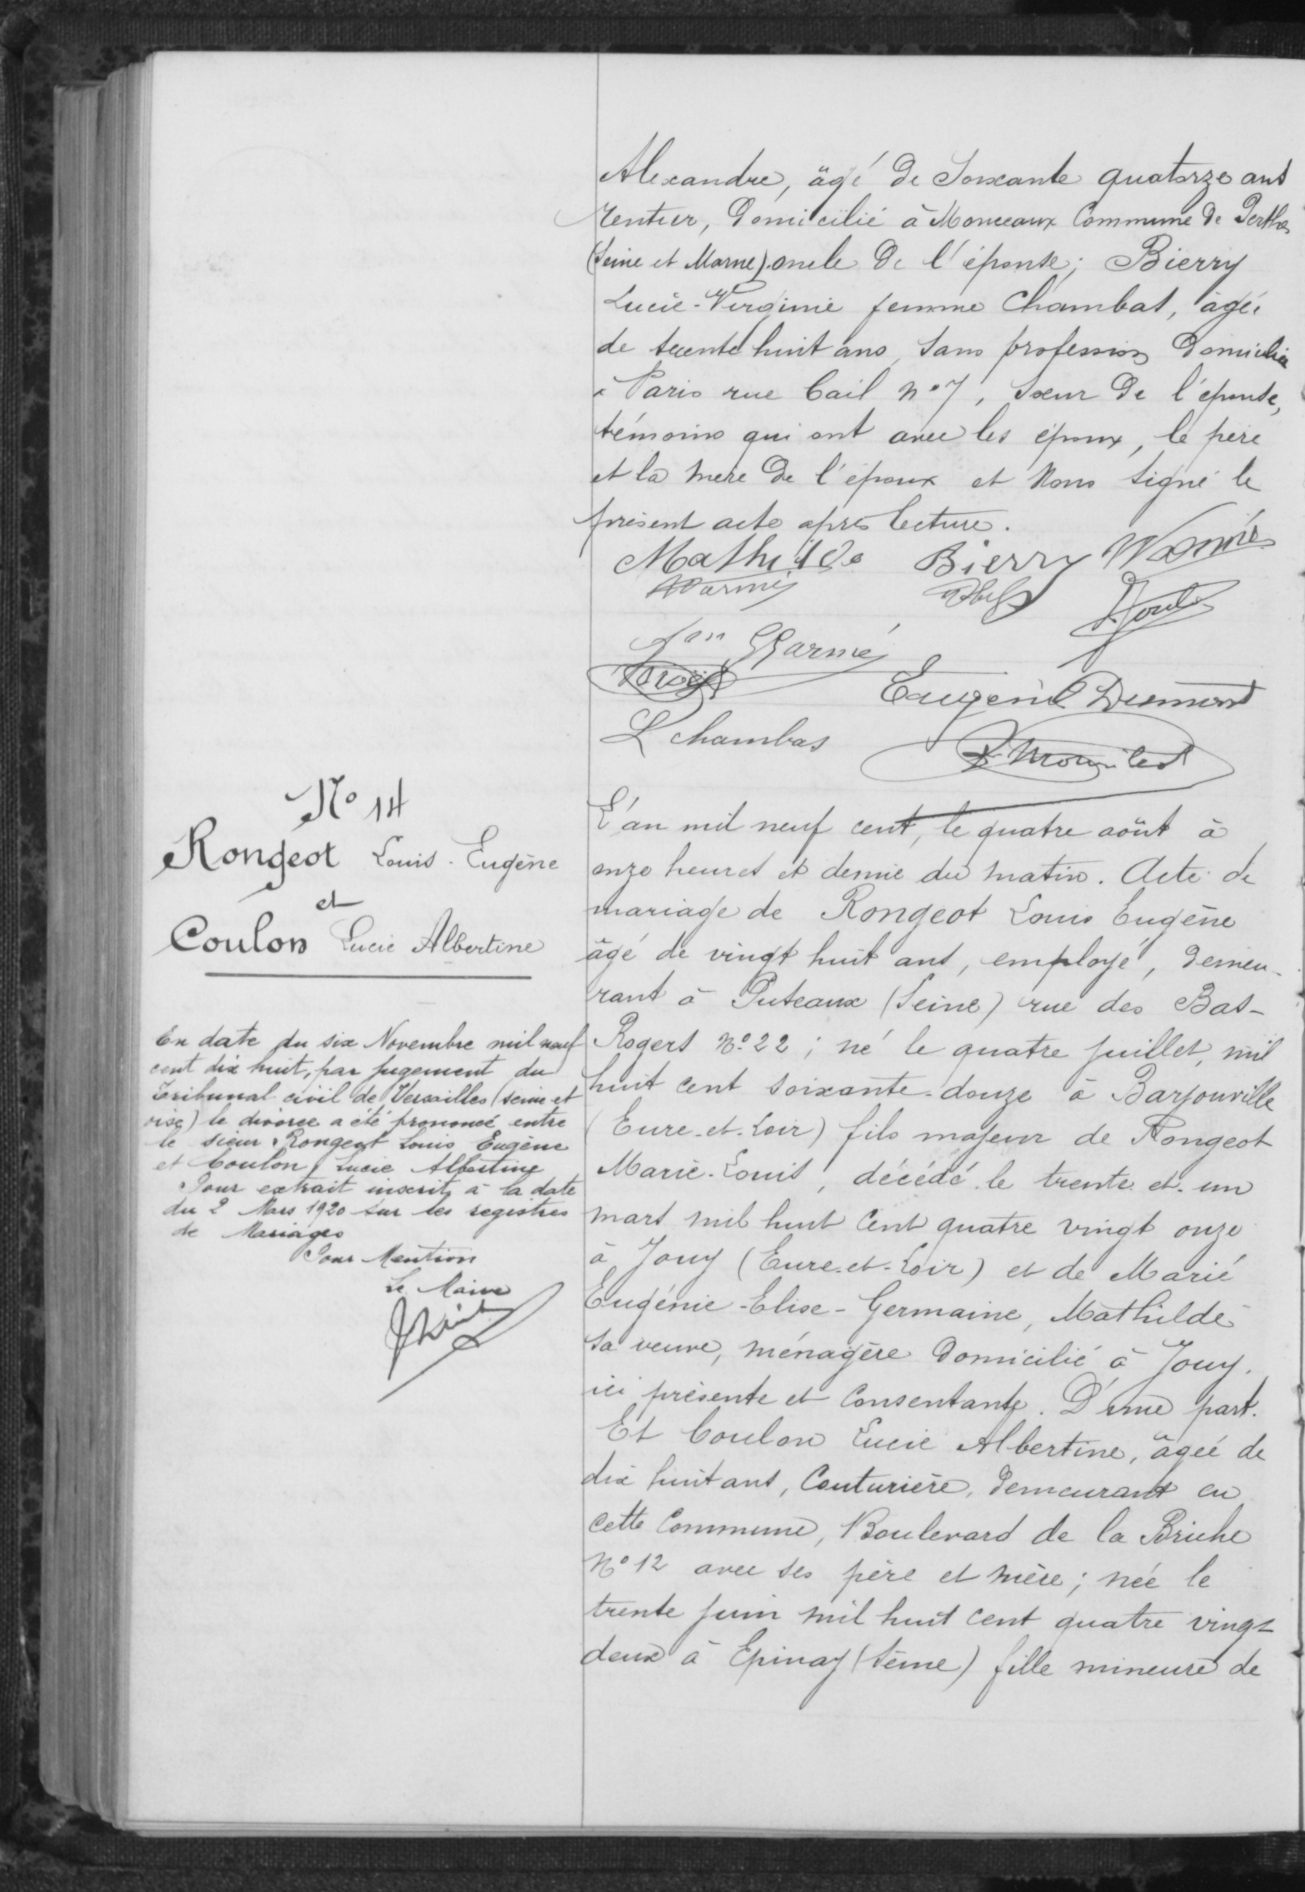Alexandre, âgé de soixante quatorze ans
rentier, domicilié à Monceaux commune de Perthe
(Seine et Marne) oncle de l'épouse; Bierry
Lucie Virginie femme Chambas, âgée
de trente huit ans, sans profession domiciliée
à Paris rue Cail n°7, soeur de l'épouse,
témoins qui ont avec les époux, le père
et la mère de l'époux et Nous signé le
présent acte après lecture.
N°14
Rongeot Louis eugène
et
Coulon Lucie Albertine
L'an mil neuf cent, le quatre août à
onze heures et demie du matin. Acte de
mariage de Rongeot Louis eugène
âgé de vingt huit ans, employé, demeu-
rant à Puteaux (Seine) rue des Bas-
Rogers N°22; né le quatre juillet, mil
huit cent soixante-douze à Barjouville
(Eure-et-Loir) fils majeur de Rongeot
Marie-Louis, décédé le trente et un
mars mil huit cent quatre vingt onze
à Jouy (Eure-et-Loir) et de Marie
Eugénie-Elise-Germaine, Mathilde
sa veuve, ménagère domicilié à Jouy
ici présente et consentante. D'une part.
Et Coulon Lucie Albertine, âgée de
dix huit ans, couturière, demeurant en
cette commune, Boulevard de la Briche
N°12 avec ses père et mère; née le
trente juin mil huit cent quatre vingt-
deux à Epinay (Seine) fille mineure de
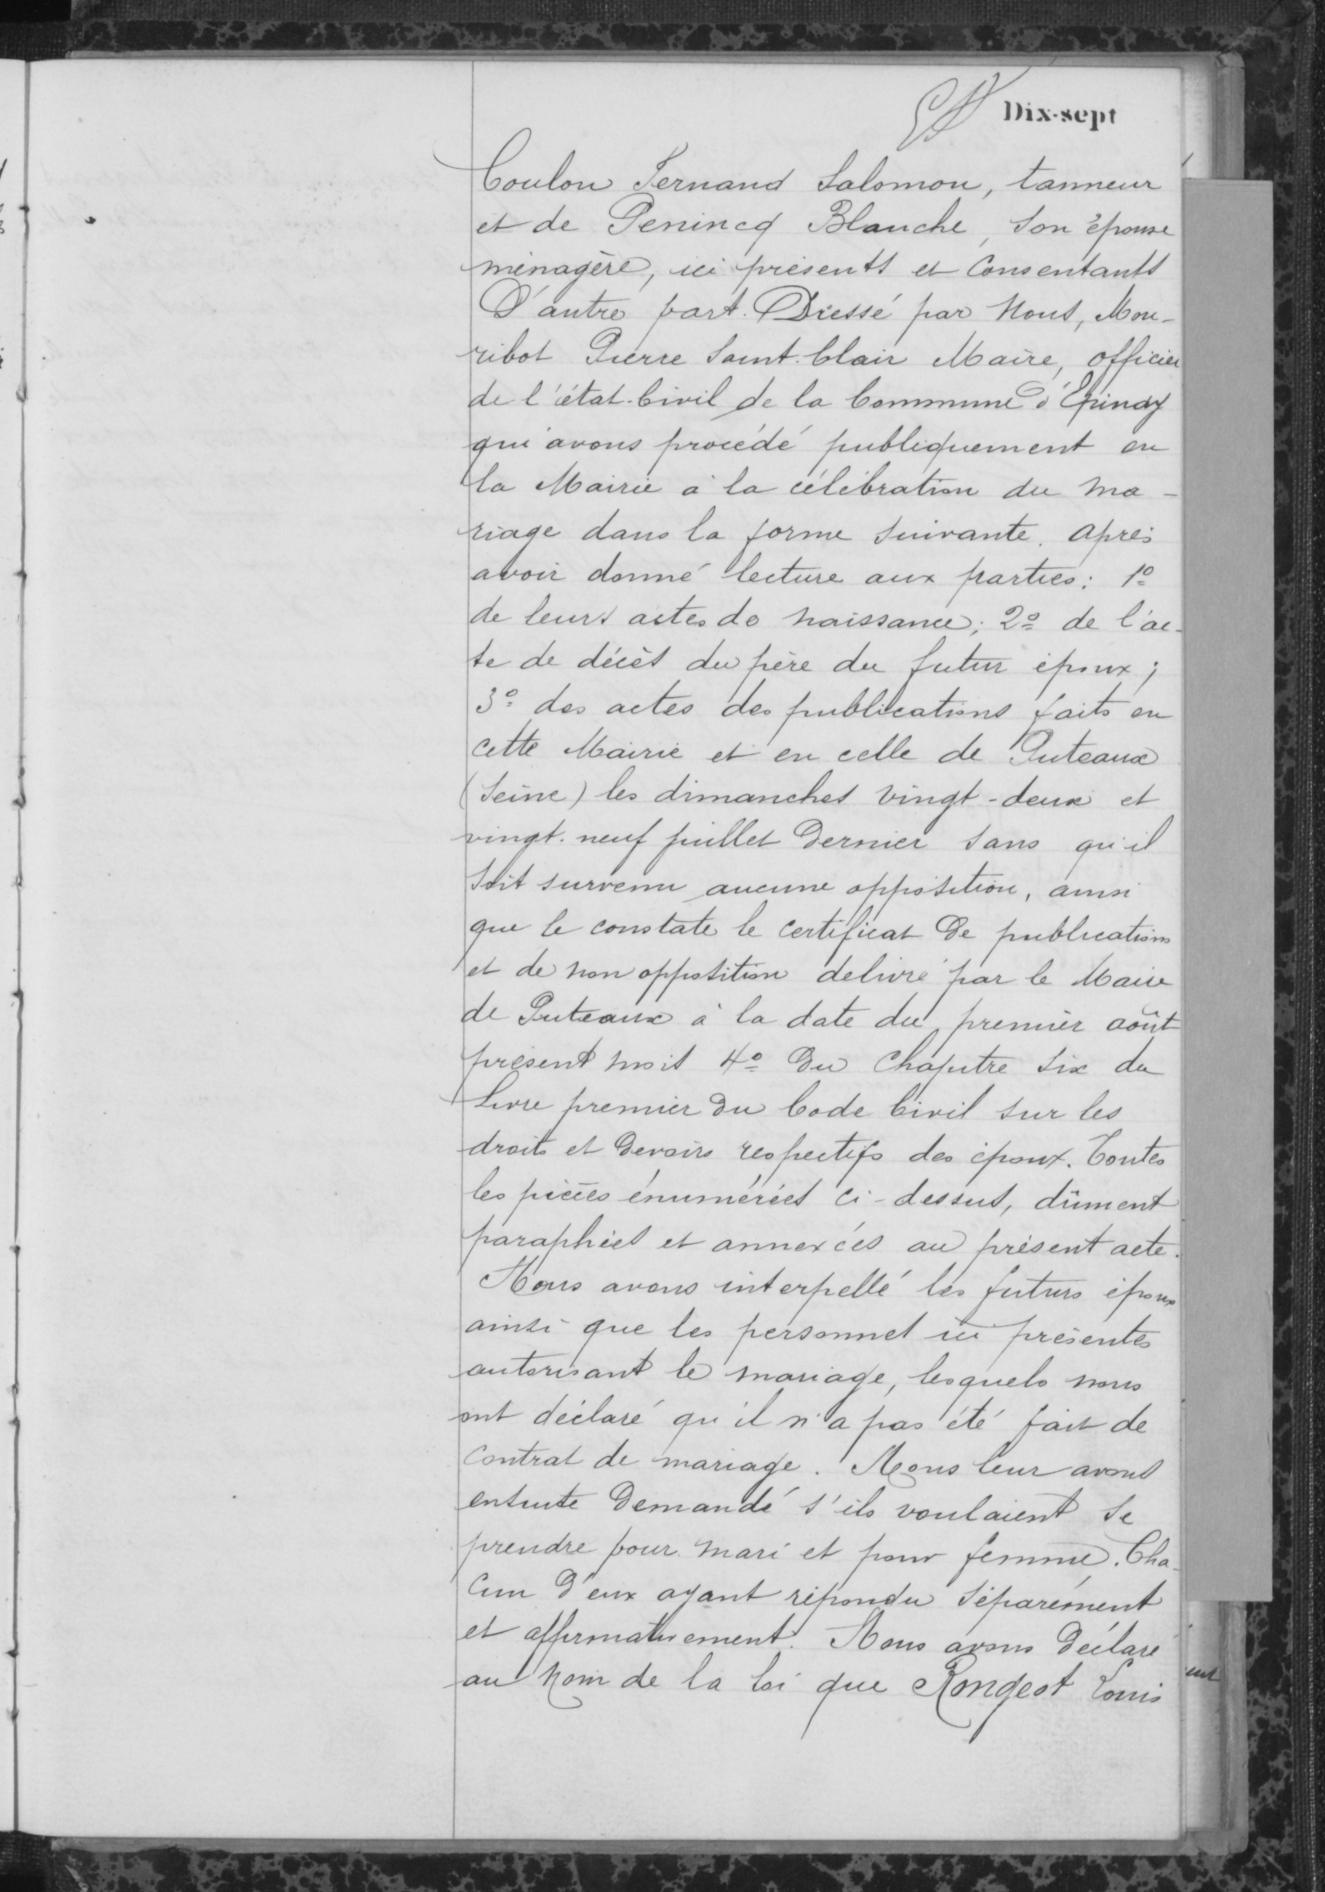Coulon Fernand Salomon, tanneur
et de Penincq Blanche, son épouse
ménagère, ici présents et consentants
D'autre part. Dressé par Nous, Mon-
ribot Pierre Saint Clair Maire, officier
de l'état Civil de la Commune d'Epinay
ui avons procédé publiquement en
la Mairie à la célébration du ma-
riage dans la forme suivante. après
avoir donné lecture aux parties: 1°/
de leurs actes de naissance; 2°/ de l'ac
te de décès du frère du futur époux;
3°: des actes des publications faits en
cette Mairie et en celle de Puteaux
(Seine) les dimanches vingt-deux et
vingt-neuf juillet dernier sans qu'il
soit survenu aucune opposition, ainsi
que le constate le certificat de publication
et de non opposition delivré par le Maire
de Puteaux à la date du premier août
présent mois 4°/ du chapitre six du
Livre premier du Code Civil sur les
droits et devoirs respectifs des époux. Toutes
les pièces énumérées ci-dessus, dûment
paraphées et annexées au présent acte
Nous avons interpellé les futurs époux
ainsi que les personnes ici présentes
autorisant le mariage, lesquels nous
ont déclaré qu'il n'a pas été fait de
contrat de mariage. Nous leur avons
ensuite demandé s'ils voulaient se
prendre pour mari et pour femme. Cha
cun d'eux ayant répondu séparément
et affirmativement. Nous avons déclaré
au nom de la loi que Rongeot Louis